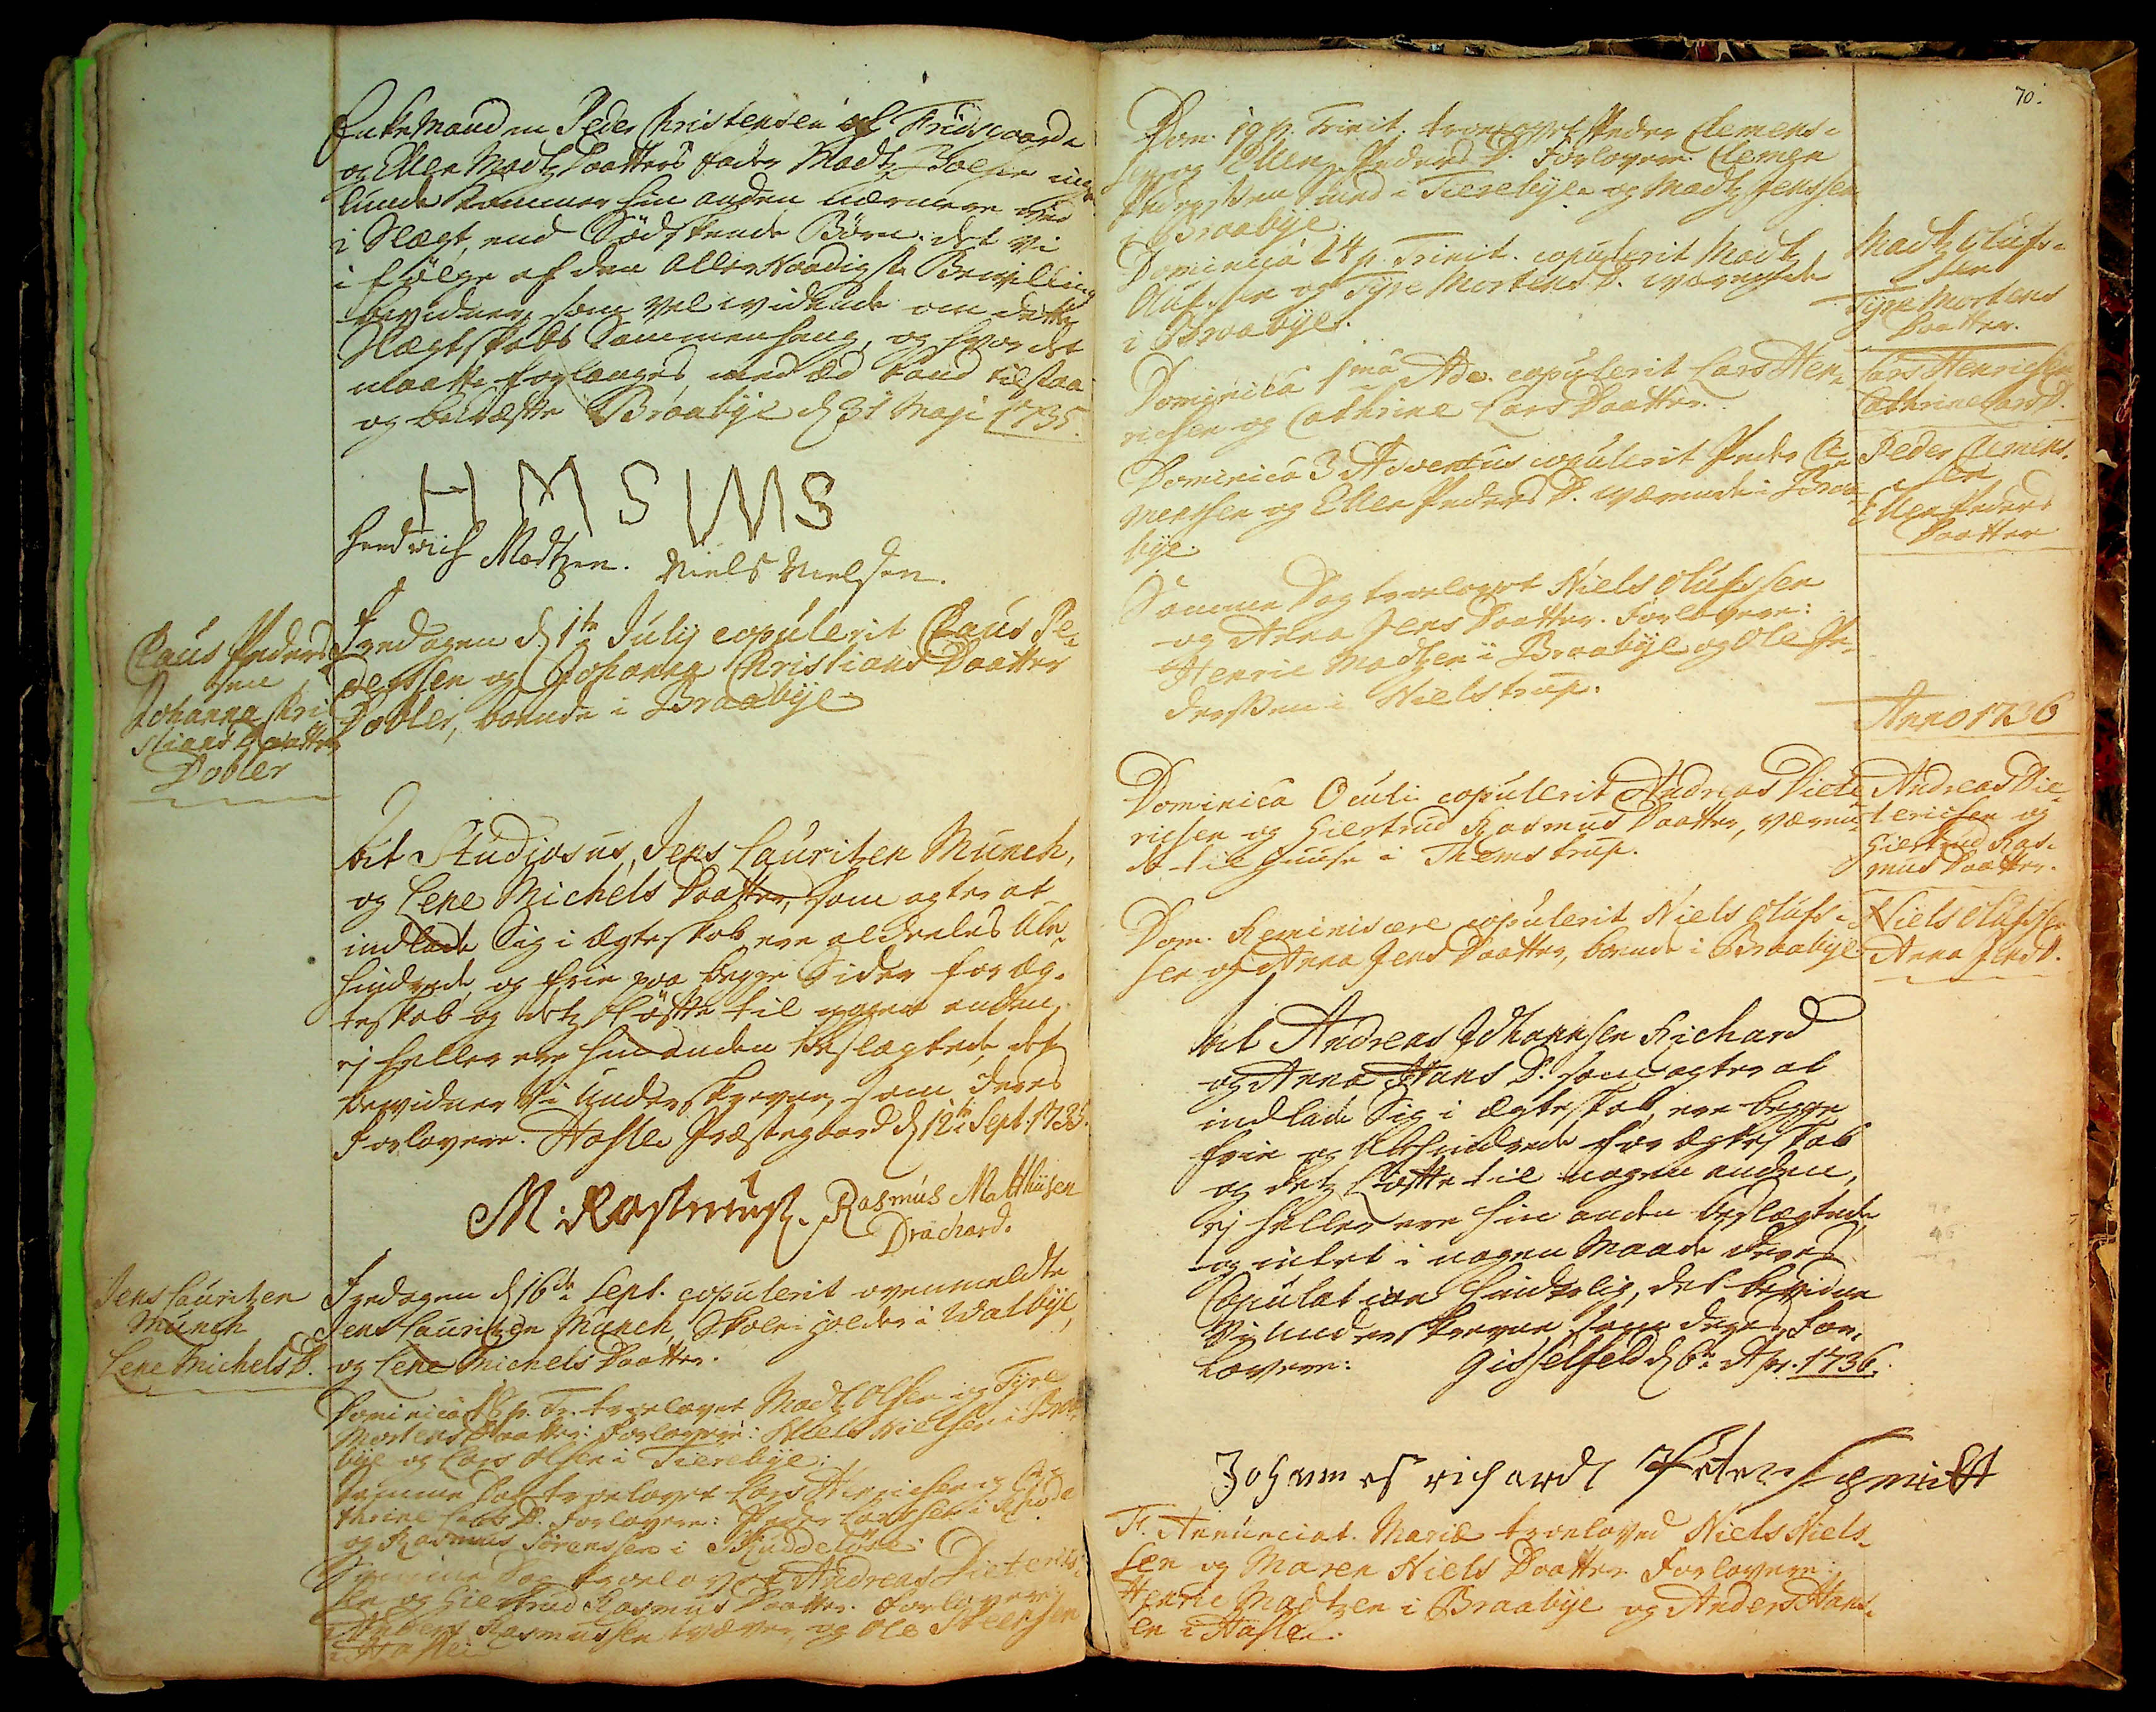Enkemanden Peder Christensen af Fridsgaarde
og Ellen Madtzdaatters Fader Madtz Boesen inge
lunde kommer hinanden nærmere ved
i Slægt end Søskende Børn det Vi
i følge af den allernaadigst Bevilling
bevidner som velvidende om dette
Slægtskabs Sammenheng, og hvor det
maatte forlanges med æd kand tilstaa
og bekræfte, Braabye d 31 May 1735.
H M S N N S 
Hendrich Madtzen. Niels Nielsen
Claus Peders
sen 
Johanne Chri
stians Daatter 
Dobler 
Fredagen d. 1ta July copulerit Claus Pe¬
derssen og Johanne Christians Daatter 
Dobler, boende i Braabye. 
At Studiosus, Jens Lauritzen Munch 
og Lene Michels Daatter, som agter at
indlade Sig i Ægteskab ere aldeeles Ube¬
hindrede og frie paa begge Sider for Æg¬
teskab og dets Løffte til nogen anden
ej heller ere hinanden beslægted, det
bevidner Vi underskrevne som deres.
Forlovere. Hasle Præstegaard d. 12te Sept. 1735.
M. Rasmus
Rasmus Matthiisen
Drachard.
Jens Lauritzen 
Munch
Lene MichelsD. 
Fredagen d.16da Sept. copulerit ovenmeldte
Jens Lauritzen Munch Skoleholder i Walbye 
og Lene Michels Daatter.
Dominica 18 p. Tr. troelovet Madtz Olsen og Tyre
Mortens Daatter: Forlovere: Niels Nielsen i Braa¬
bye og Lars Olsen i Tierebye.
Samme Dag troelovet Lars Henricsen og Ca
thrine LarsD. Forlovere: Peder Larssen i Rhode##
og Rasmus Sørensen i Skuddeløse.
Samme Dag troelovet Andreas Dieteric##¬
sen og Giertrud Rasmus Daatter. Forlovere:
Anders Rasmussen Væver, og Ole Steensen
i Hasle.
Den 19 p. Trinit. troelovet Peder Clemens¬
sen og Ellen Peders D. Forlovere: Clemen
## ## Smed i Tierebye og Madtz Jenssen
i Braabye
70.
Dominica 24 p. Trinit. copulerit Madtz
Olufssen og Tyre MortensD. værende
i Braabye. 
Madtz Olufs¬
sen 
Tyre Mortens
Daatter.
Dominica 1ma Adv. copulerit Lars Hen¬
ricsen og Cathrine Lars Daatter.
Lars Henrichsen
Cathrine LarsD.
Dominica 3 Adventus copulerit Peder Cle##¬
menssen og Ellen Peders D. værende i Braa¬
bye. 
Peder Clemens¬
sen
Ellen Peders
Daatter
Samme Dag troelovet Niels Olufssen
og Anna Jens Daatter. Forlovere:
Henric Madtzen i Braabye og Ole Pe¬
derssen i Nielstrup. 
Dominica Oculi: copulerit Andreas Diete
ricsen og Giertrud Rasmus Daatter, værende
til Huuse i Themstrup.
Anno 1736
Andreas Die¬
tericsen og 
Giertrud Ras¬
mus Daatter.
Dom. Reminiscere copuleret Niels Olufs¬
sen og Anna Jens Daatter, boende i Braabye.
Niels Olufssen
Anna JensD.
At Andreas Johannsen Richard
og Anna Hans D. som agter at
indlade Sig i Ægteskab ere begge
frie og ubehindrede for Ægteskab
og dets Løftte til nogen anden,
ej heller ere hin anden beslægtede
og intet i nogen maade deres
Copulation hinderlig, det bevidner
Vi underskrevne som deres For¬
lovere: 
Gisselfeld d. 6ta Apr. 1736
Johannes richardt Peter Schmitt
Fr. Annunciati Mariæ troeloved Niels Niels¬
sen og Maren Niels Daatter. Forlovere:
Henric Madtzen i Braabye og Anders Hans¬
sen i Hasle.
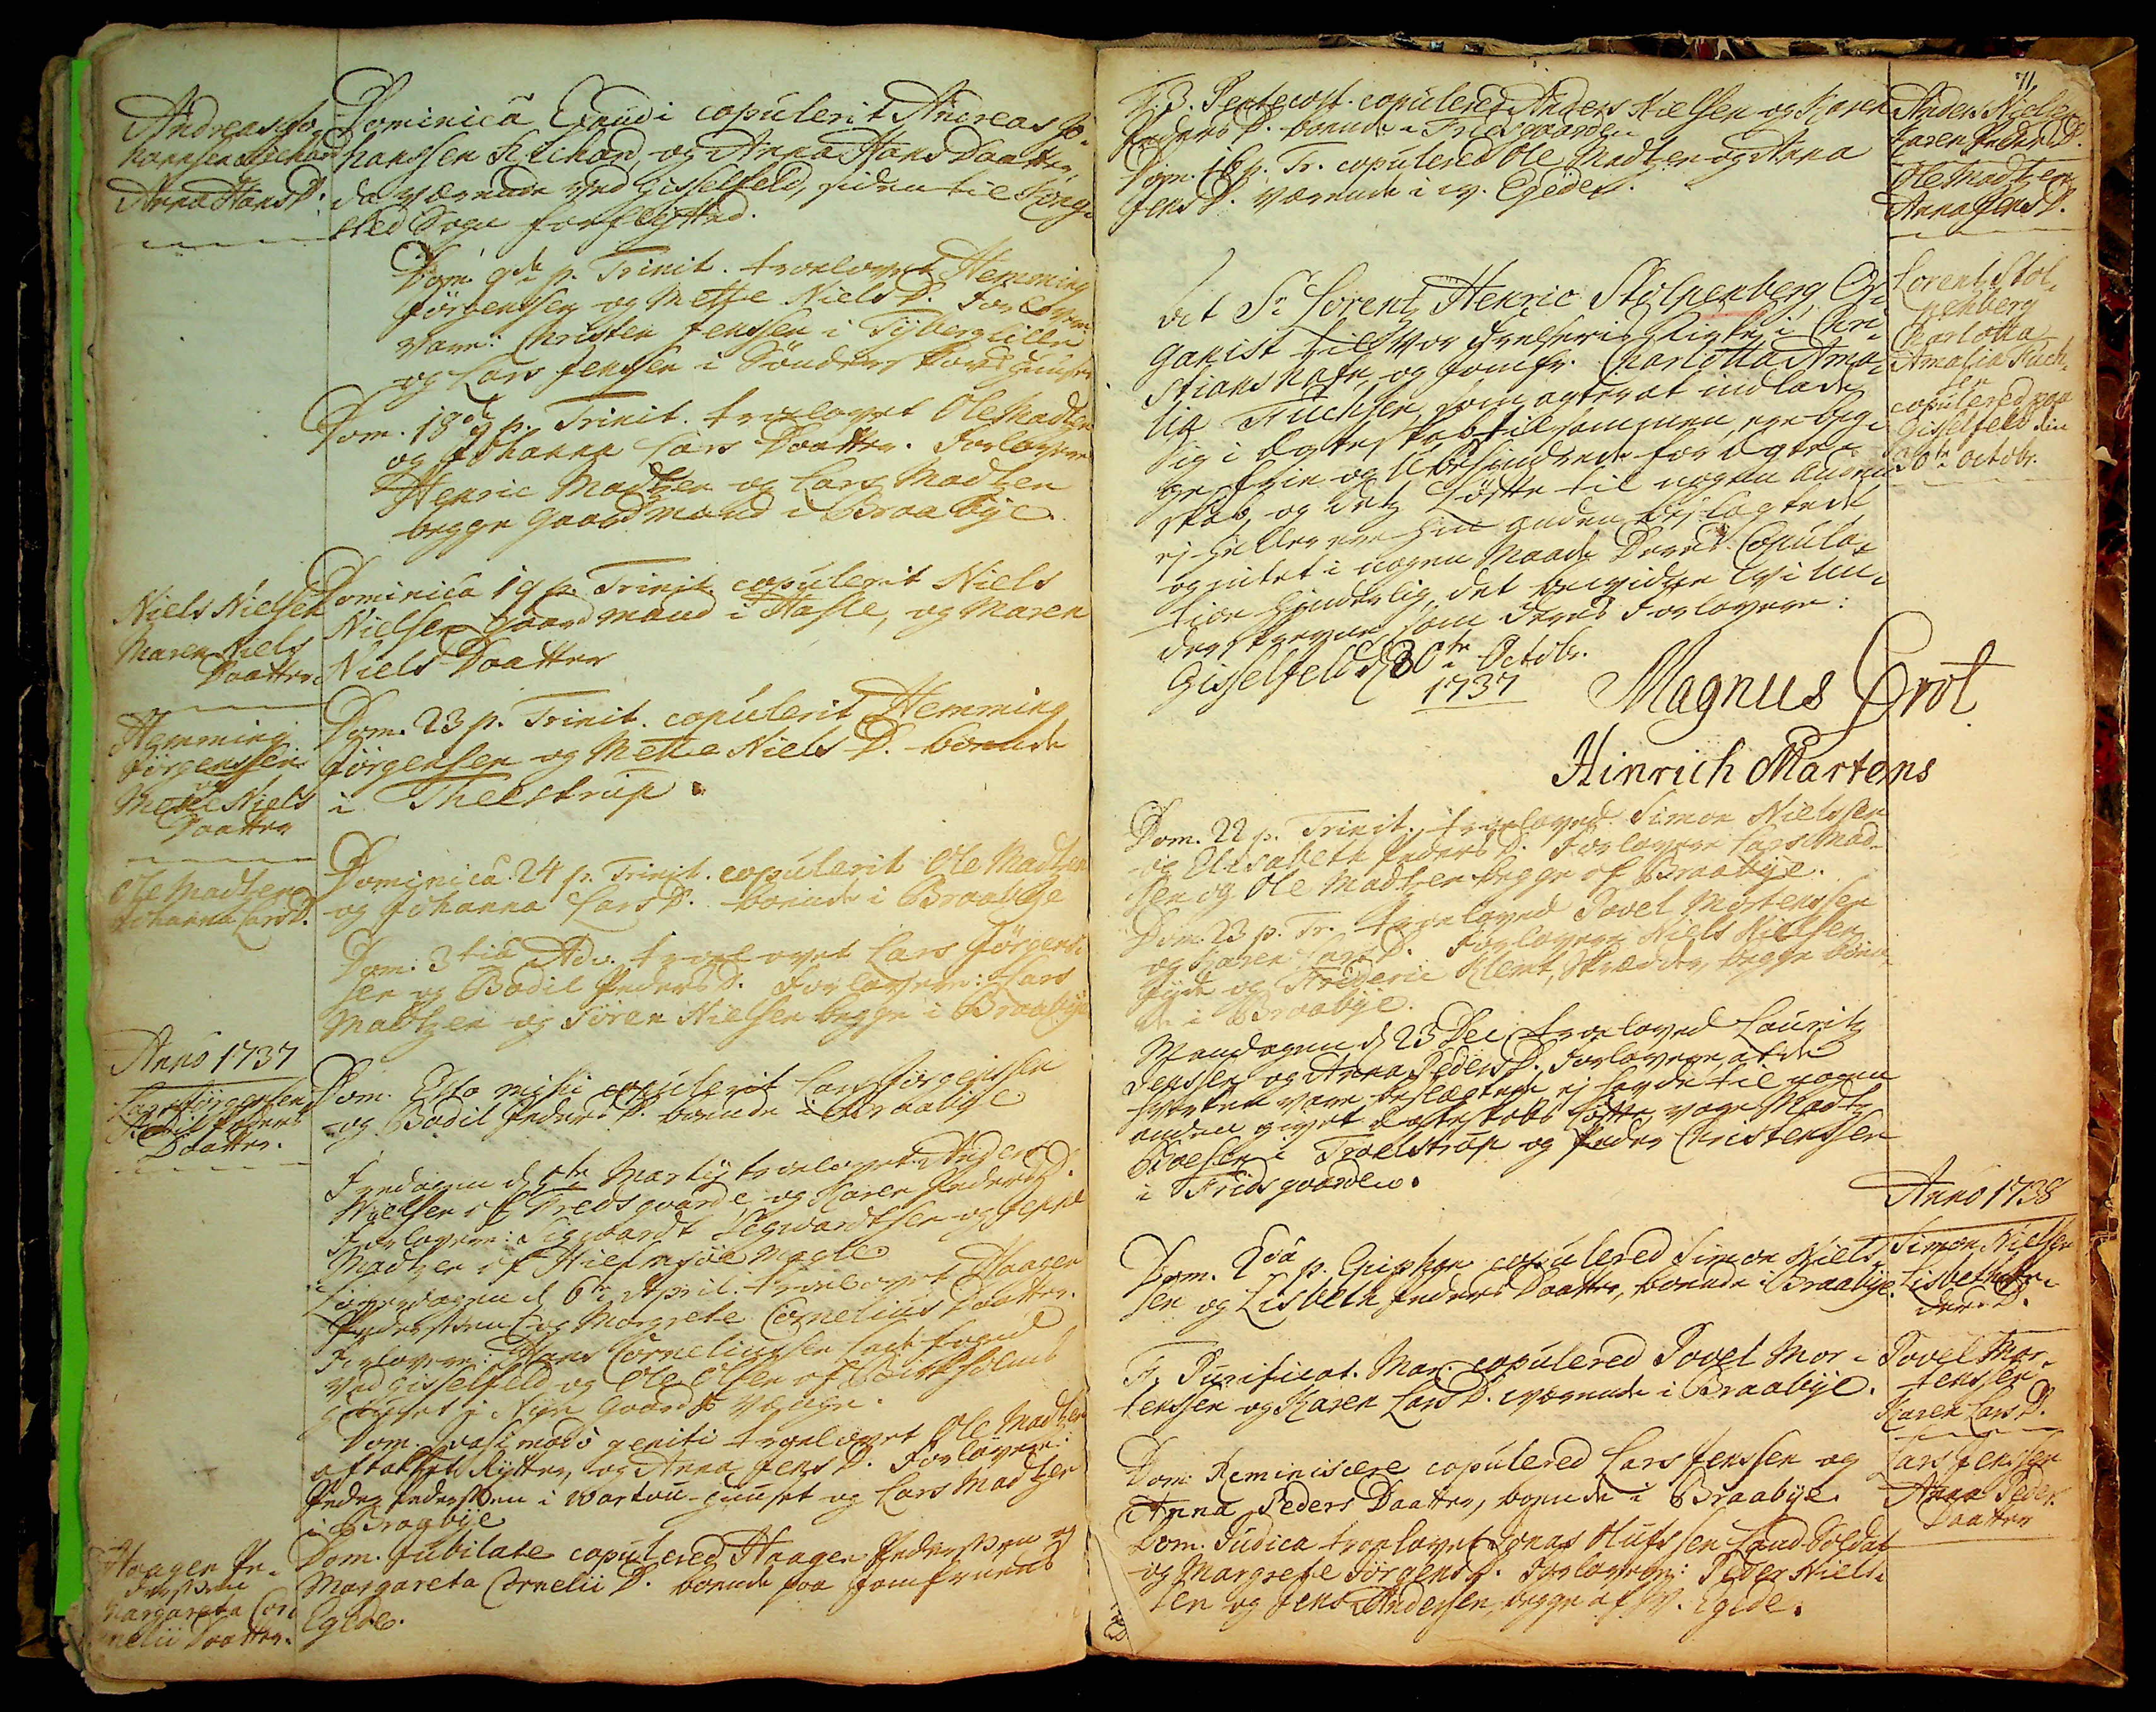Andreas Jo¬
hannsen Richard 
Anna HansD. 
Dominica Exaudi copulerit Andreas Jo¬
hanssen Richard, og Anna Hans Daatter,
da værende ved Gisselfeld, siden til Kong¬
sted Sogn forflytted.
Dom. 9de p. Trinit. troelovet Hemming
Jørgenssen og Mette NielsD. Forlovere
vare: Christen Jenssen i Tyberglille
og Lars Jenssen i Sønder Skovs Huuset.
Dom. 18 de p. Trinit. troelovet Ole Madtzen
og Johanne Lars Daatter. Forlovere:
Henric Madtzen og Lars Madtzen
begge Gaardmænd i Braabye.
Niels Nielsen 
Maren Niels
Daatter. 
Dominica 19 p. Trinit. copulerit Niels
Nielsen Gaardmand i Hasle, og Maren
Niels Daatter. 
Hemming 
Jørgenssen
Mette Niels
Daatter 
Dominica 23 p Trinit. copulerit Hemming
Jørgensen og Mette NielsD. - boende
i Theestrup.
Ole Madtzen 
Johanna LarsD. 
Dominica 24 p. Trinit. copulerit Ole Madtzen
og Johanna LarsD. boende i Braabye. 
Dom. 3tia Adv. troelovet Lars Jørgens¬
sen og Bodil PedersD. Forlovere: Hans 
Madtzen og Søren Nielsen begge i Braabye. 
Anno 1737
Lars Jørgenssen
Bodil Peders
Daatter 
Dom Esto mihi copulerit Lars Jørgenssen
og Bodil PedersD. boende i Braabye.
Fredagen d. 1te## Marty troelovet Anders
Nielsen i Fredsgaarde, og Karen PedersD.
Forlovere: Eggardt Sigwardtsen og Jeppe
Madtzen i Hielmsømagle.
Løverdagen d. 6ta## April troelovet Haagen
Pederssen og Margrete Cornelius Daatter.
Forlovere: Hans Corneliussen Ladefoged
ved Gisselfeld og Ole Olsen af Birck##holm
Huuset i Nye Gaards Vænge##.
Dom Quasimodo geneti troelovet Ole Madtzen
aftakket Rytter og Anna JensD. Forlovere:
Peder Pederssen i Wartou Huuset og Lars Madtzen
i Braabye.
Haagen Pe¬
derssen
Margareta Cor
nelii Datter.
Dom Jubilate copulered Haagen Pederssen og
Margareta Conelii D. boende paa Jomfruens
Egede. 
F. 3 Pentecoste. copulered Anders Nielsen og Karen
Peders D. boende i Fridsgaarde.
71.
Anders Nielsen 
Karen PedersD. 
Dom. 16 p. Tr. copulered Ole Madtzen og Anna
Jens D. værende i W.Egede.
Ole Madtzen 
Anna Jens D.
At SrLorentz Henric Stolpenberg, Or¬
ganist til Vor Frelseris Kirke i Chri¬
stians Hafn, og Jomfru Charlotta Ama¬
lia Fuchsen, som agter at indlade
sig i Ægteskab til sammen ere beg¬
ge, frie og ubehindrede for Ægte¬
skab og dets Løftte til nogen anden
ej heller ere hinanden beslægtede
og intet i nogen Maade Deres Copula¬
tion hinderlig, det bevidner Vi un¬
derskrevne som deres Forlovere:
Gisselfeld d. 30te Octobr.
1737
Magnus Grot
Hinric Martens
Lorentz Stol¬
penberg
Charlotta 
Amalia Fuch¬
sen 
copulered paa
Gisselfeld den
30ta October
Dom. 22 p. Trinit. troeloved Simon Nielssen
og Elisabeth PedersD. Forlovere Lars Mad¬
tzen og Ole Madtzen begge af Braabye.
Dom. 23 p. Tr troeloved Povel Mortenssen
og Karen Lars D. Forlovere Niels Nielsen
Jyde og Frideric Klemt Skrædder begge boen¬
de i Braabye. 
Mandagen d. 23 Dec. troeloved Lauritz
Jenssen og Anna Peders D. Forlovere, at de
hverken## være beslægtede ej havde til nogen
anden og givet Ægteskabsløftte, vare. Madtz
Boesen i Troelstrup og Peder Christenssen
i Fridsgaarde.
Dom. 2da Epiphan copulered Simon Niels¬
sen og Lisbeth Peders Daatter, boende i Braabye.
Anno 1738
Simon Nielssen 
Lisbeth Pe¬
ders D.
F. Purificat. Mar. copulered Povel Mor¬
tenssen og Karen Lars D, værende i Braabye.
Povel Mor-
tenssen 
Karen Lars D.
Dom Reminiscere copulered Lars Jenssen og
Anna Peders Daatter, boende i Braabye.
Lars Jenssen 
Anna Peders
Daatter 
Dom Judica troelovet Jonas Olufssen Land-Soldat
og Margrete Jørgens D. Forlovere: Peder Niels¬
sen og Jens Andersen, begge af W. Egede.